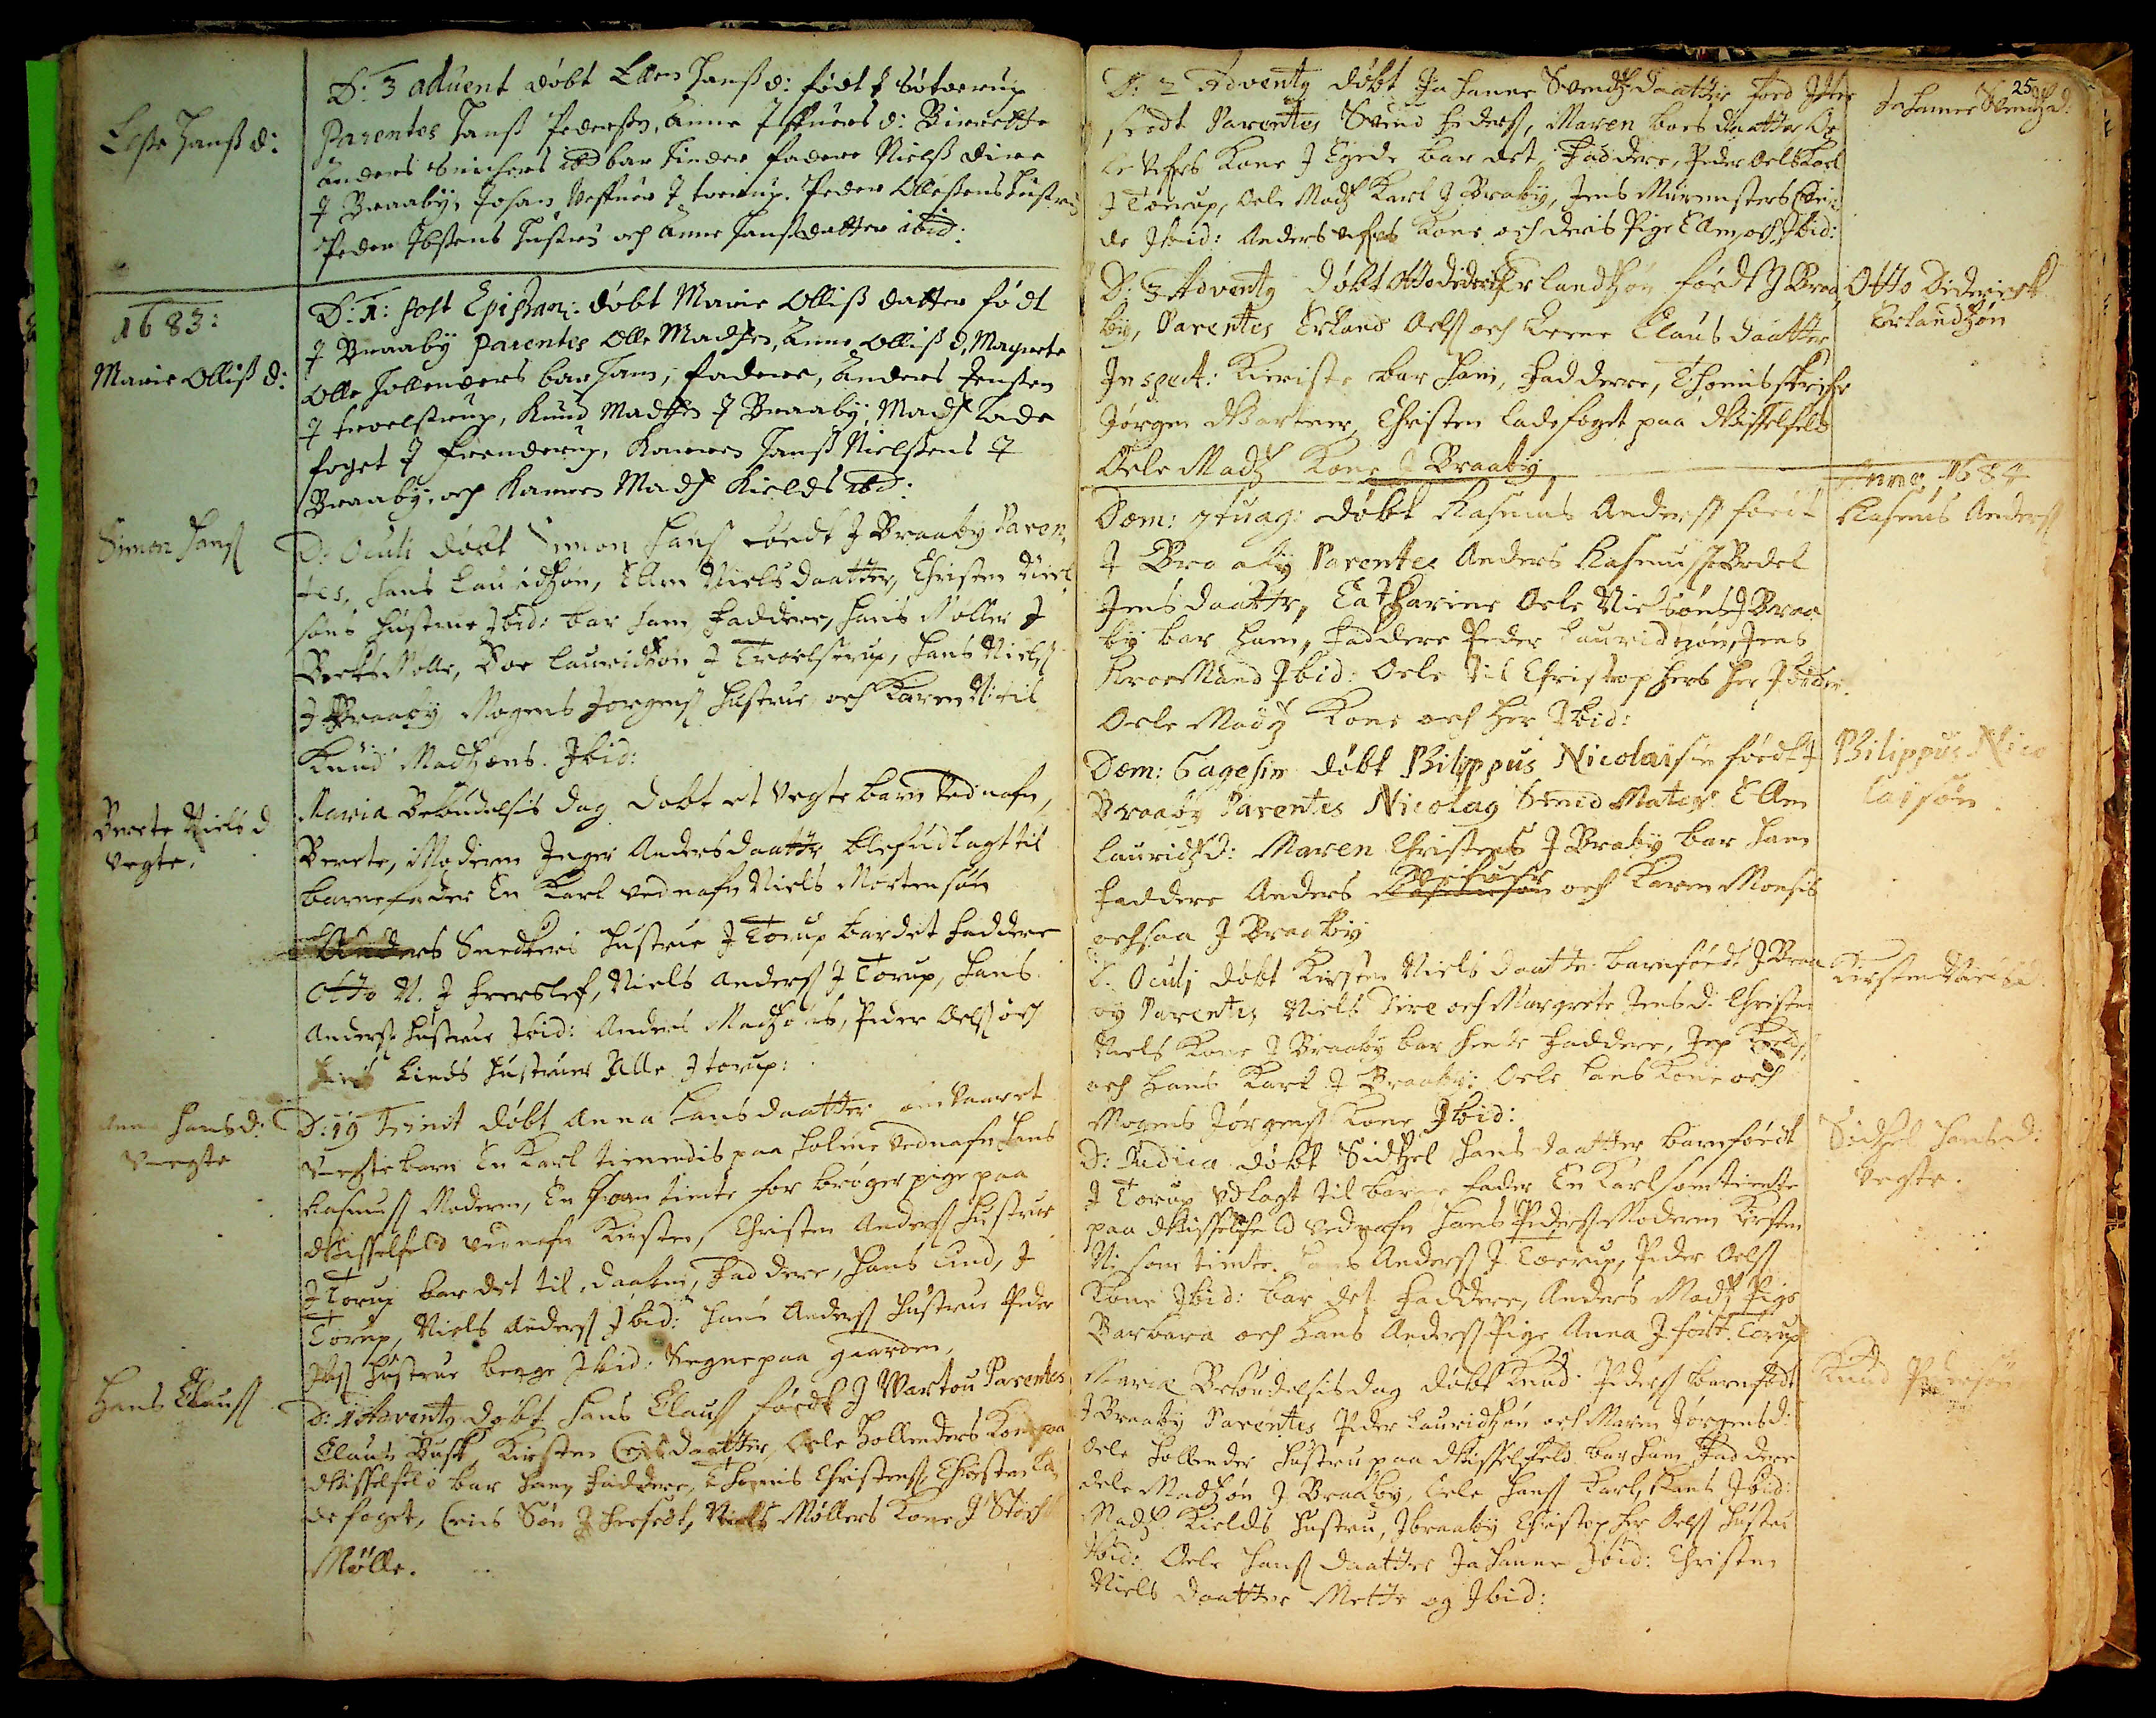Ellen Hans d:
D: 3 Aduent døbt Ellen Hansd: født J Søtoerup
Parentes Hans Pedersen, Anne Jffuors d: Birrette
Anders Snichers ibd. bar hinder, Fadere Niels Dire
J Braaby, Johan Veffuer J Toerup. Peder Ollesens Hustru,
Peder Jbsens Hustru och Anne Hansdatter ibd:
1683:
Marie Ollis d: 
D: 1: post Epiphan: døbt Marie Ollis datter født
i Braaby Parentes Olle Madsen, Anne Ollis d:. Magrete
Olle Hollenders bar ham; Fadere, Anders Jensen
J Troelstrup, Knud Madsen i Braaby, Mads Lade
foget i Frenderup, Karren Hans Nielsens J
Braaby och Karren Mads Kields ibd.
Simon Hans 
D: Oculi døbt Simon Hans føbt J Braaby. Paren¬
tes, Hans Lauridsøn, Ellen Nielsdaatter, Christen Niel¬
søns Hustru Jbid: bar ham, Faddere, Hans Møller J
Becks Mølle, Boe Lauridsøn J Troelstrup, Hans Niels
J Braaby, Mogens Jørgens Hustru, och Karren N: til
Knud Madsens Jbid:
Berete Niels d: 
Uegte 
Maria Bebudelses dag døbt et Uegte barn ved nafn, 
Berete, Moderen Jnger Andersdaatter blef udlagt til 
Barnefader En Karl ved nafn Niels Mortensøn
Anders## Snedkers Hustru J Torup bar det, Fadder
Otte N. J Frerslef, Niels Anders J Torup, Hans
Anders Hustru Jbid: Anders Madsøns, Peder Oelsøns,
Hans Kiels## Hustru alle J Torup:
Anna Hansd: 
Uegte
D: 19 Trinit døbt Anna Hansdatter en## vaaret
Uegte barn. En karl tienndis paa Holme ved nafn Hans
Rasmus Moderen, En som tiente for brøger pige paa
Gisselfeld ved nafn Kirsten, Christen Anders Hustrue
J Torup bar det til daaben, Faddere, Hans Lind J
Torup, Niels Anders Jbid: Hans Anders Hustrue Peder
Jbs Hustrue begge Jbid: Segne paa gaarden.
Hans Clausen 
D: 1 Adventz døbt Hans Claus født J Vartou. Parentes:
Claus Busk, Kirsten Ceisdaatter, Oele Hollenders Kone paa
Gisselfeld bar ham, Faddere, Thomis Christens, Christen La¬
defoget, Ceis Søn J Heesedt, Niels Møllers Kone J Stochs
Mølle.
D: 2 Adventz døbt Johanne Svendsdaatter føed J Hee
sedt. ## Parentes Svend Peders, Maren Boesdaatter, Ol
le Veffers Kone J Egede bar det, Faddere, Peder Oels Karl
J Toerup, Oels Mads Karl J Braby, Jens Murmesters Quin¬
de Jbid: Anders Vefvers Kone och deris Pige Ellen Ols Jbd:
25
Johanne Svendsd:
D: 3 Adventz døbt Otto Diderich Erlandsøn født J Braa
by, Parentes Erland Oels och Leene Claus daatter
Inspect: Kieriste bar ham, Faddere, Thomis skriffr,
Jørgen Gartner, Christen Ladefoget paa Gisselfeld
Oele Mads kone J Braaby.
Otto Diderick
Erlandszøn
Dom: 7tuag: døbt Rasmus Anders føedt
J Braaby, Parentes: Anders Rasmus, Bodel
Jensdatter, Catharine Oele Nielsøns J Braa
by bar ham, Faddere Peder Lauridzøn, Jens
KroesMand Jbid: Oele til Christophers her Jbidum
Oele Madz Kone och her Jbid:
Anno: 1684
Rasmus Andersen 
Dom: 6 agesim døbt Philippus Nicolaisøn føedt J 
Braaby Parentes Nicolag Smid Mater: Ellen
Lauridsd: Maren Christens J Braby bar ham
Viefurs ##
Faddere Anders Viefurs ## och Karen Monsis
ochsaa J Braaby
Philippus Nico
laisøn
D: Oculi døbt Kirsten Nielsdaatter barnføedt J Braa
by Parentes Niels Dire och Margrete Jens d: Christen
Niels kone J Braaby bar hinde Faddere, Jep Kjelds.
och hans Karl J Braaby: Oele Hans Kone och
Mogens Jørgens Kone Jbid:
Kirsten Nielsd:
D: Judica Sidsel Hansdaatter barnføedt
J Torup Udlagt til barne fader En Karl som tiente
paa Gisselfeld ved nafn Hans Pedersen, Moderen Kirsten
N: som tiente Hans Anders J Toerup, Peder Oels
Kone Jbid: bar det Faddere Anders Mads Pige
Barbara och Hans Anders Pige Anna J Søet## Torup.
Sidzel Hans d: 
Uegte
Mariæ Bebudelsisdag døbt Knud Peders barnefødt
J Braaby Parentes Peder Lauridsøn och Maren Jørgensd:
Oele Hollender Hustru paa Gisselfeld bar ham Faddere
Oele Madsøn J Braaby, Oele hans karl, Bent Jbid:
Madz Kields Hustru i Braaby Christopher Oels Hustru
Jbid: Oele Hans daatter Johanne Jbid: Christen
Niels daatter Mette og Jbid:
Knud Pedersøn